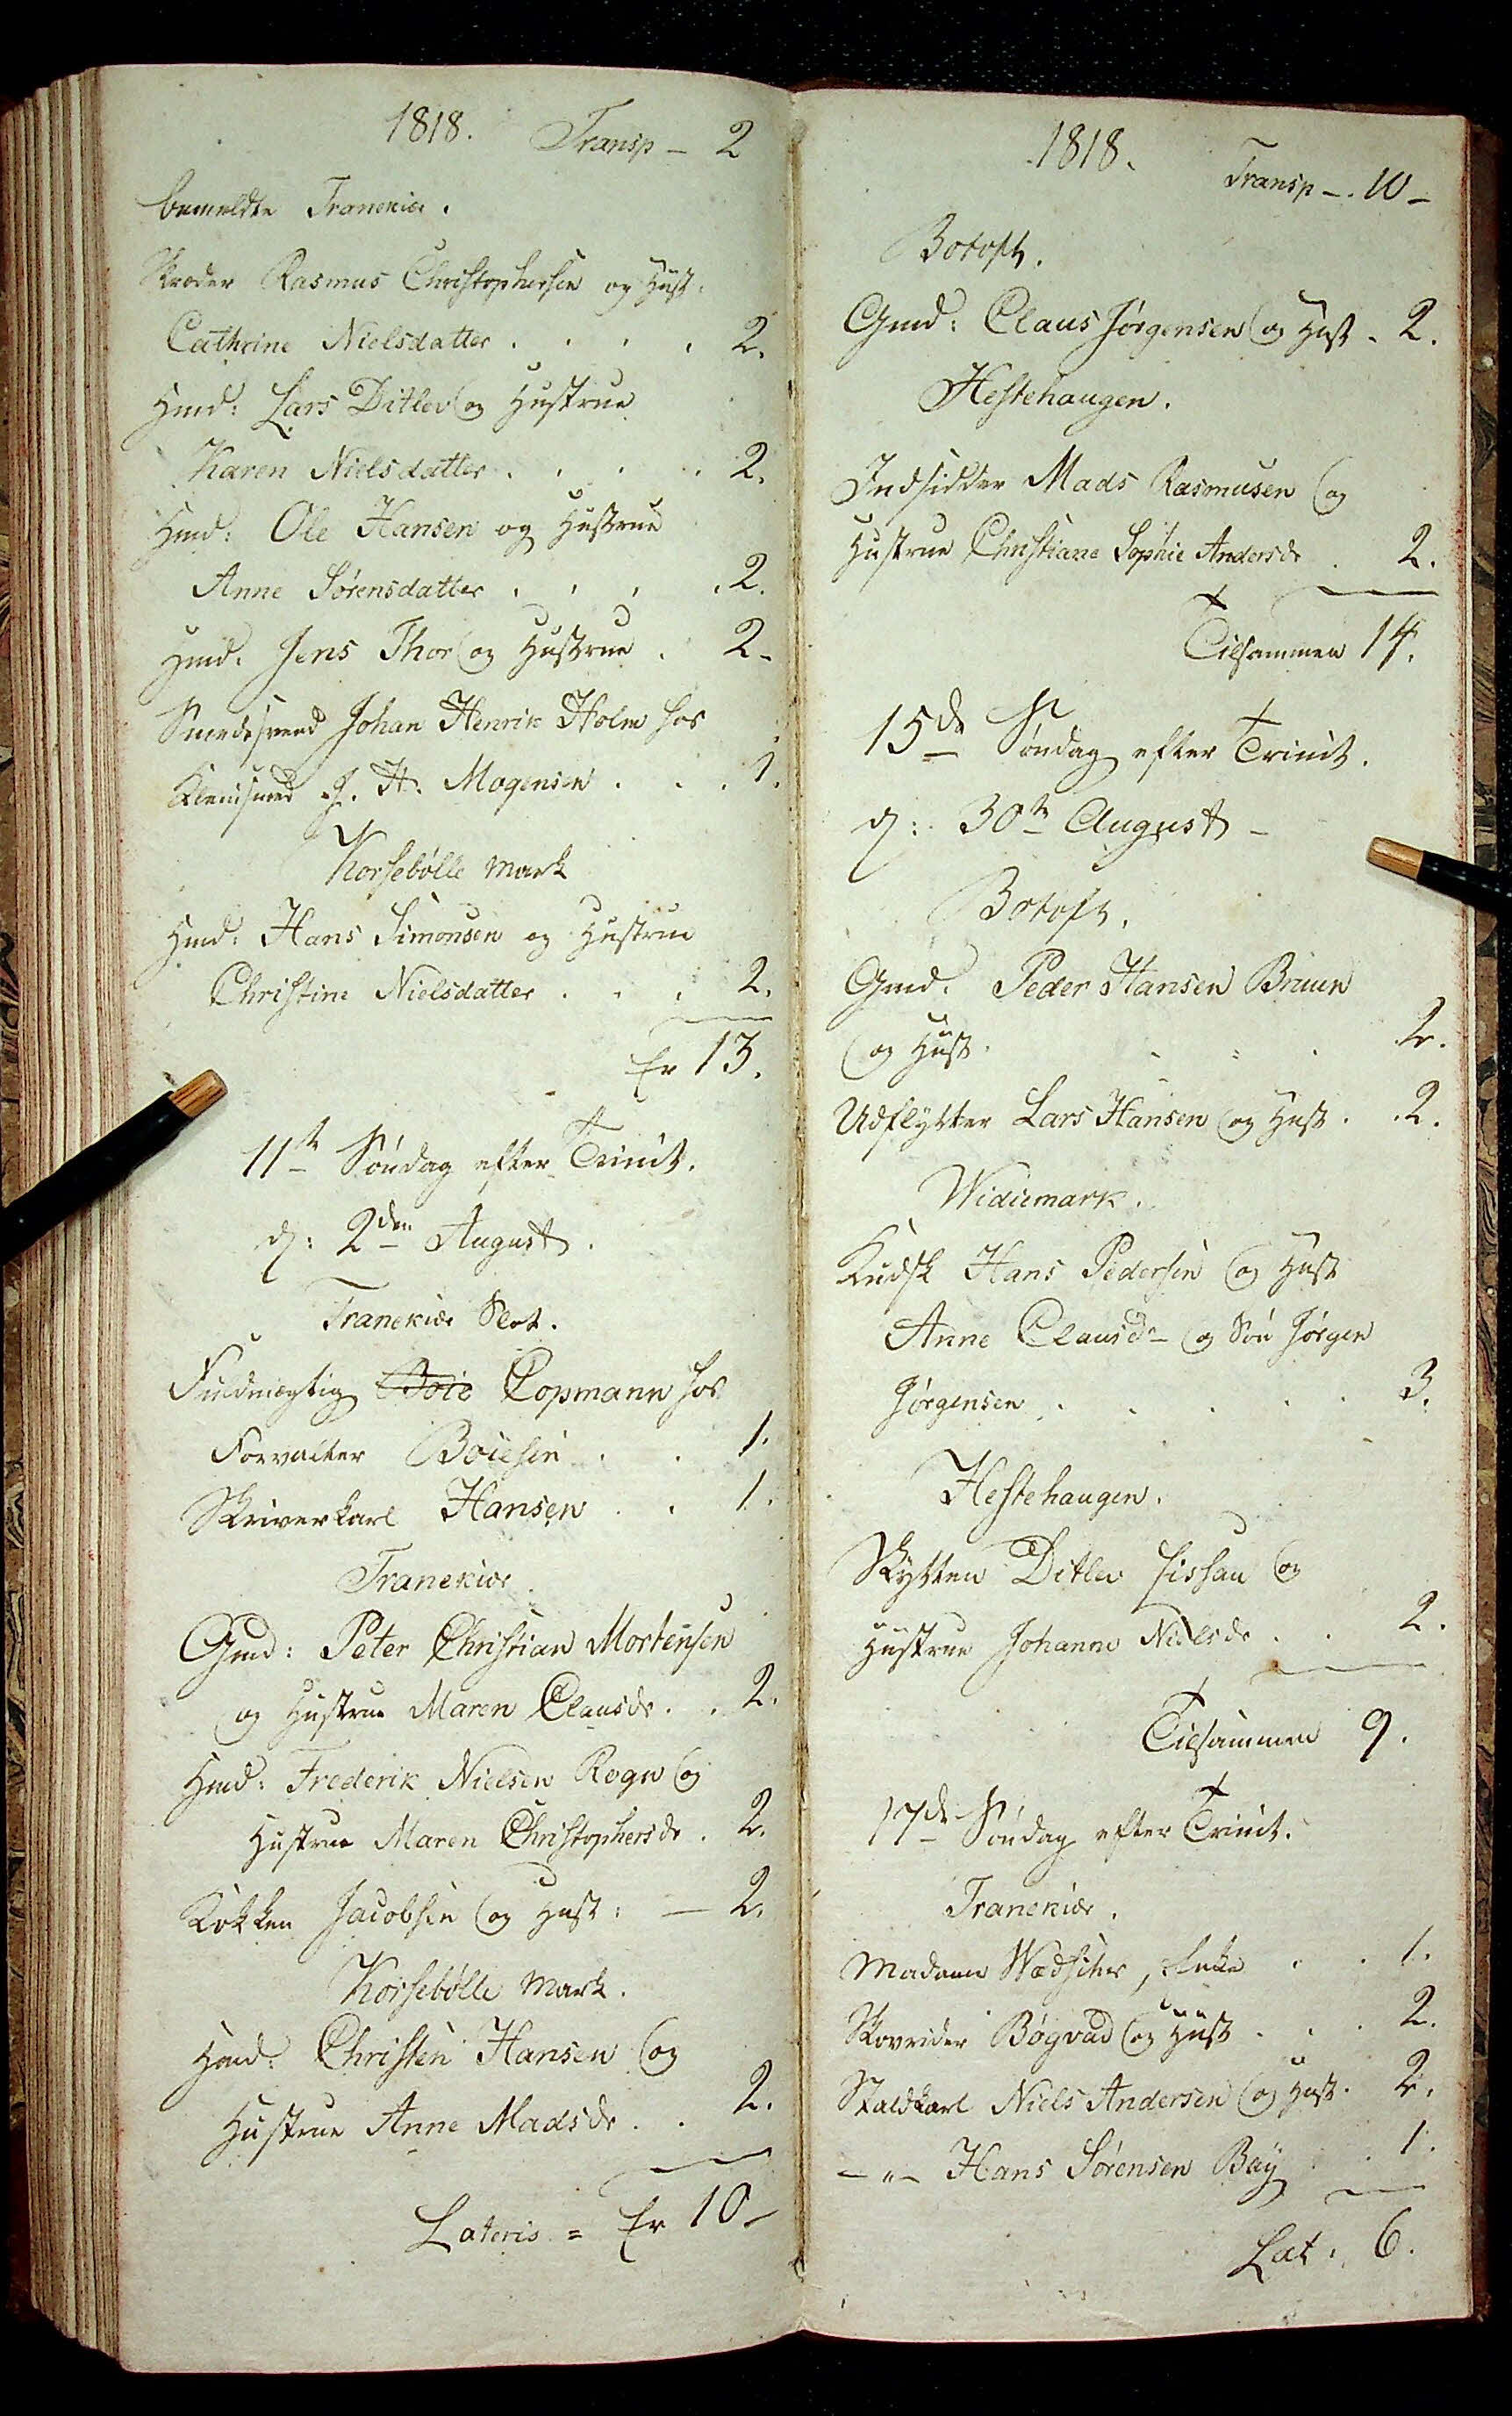1818.
Transp - 2
bemeldte Tranekiær.
Skræder Rasmus Christophersen og Hust:
Cathrine Nielsdatter . . . . 2.
Hmd: Lars Ditlev og Hustrue
Karen NielsDatter . . . . 2.
Hmd: Ole Hansen og Hustrue
Anne Sørensdatter . . . 2.
Hmd: Jens Thor og Hustrue . 2.
Smedsvend Johan Henrik Holm hos
Kleinsmed J. H. Mogensen . . .  1.
Korsebølle Mark
Hmd: Hans Simonsens og Hustrue
Christine Nielsdatter . . . 2.
Er 13.
11te Søndag efter Trinit.
d: 2den August
Tranekiær Slot.
Fuldmægtig Boie Copmann hos
Forvalter Boiesen . . 1.
Skriverkarl Hansen . . 1.
Tranekiær
Gmd: Peter Christian Mortensen
og Hustrue Maren Clausdr . . 2.
Hmd: Frederik Nielsen Rogn og
Hustrue Maren Christophersdr . 2.
Kokken Jacobsen og Hust: - 2.
Korsebølle Mark.
Hmd: Christen Hansen og
Hustrue Anne Madsdr . . 2.
Lateris = Er 10-
1818.
Transp - . 10-
Botoft..
Gmd: Claus Jørgensen og Hust . 2.
Hestehaugen.
Indsidder Mads Rasmusen og
Hustrue Christiane Sophie Andersdr . 2.
Tilsammen 14.
15de Søndag efter Trinit.
d: 30te August
Botoft.
Gmd. Peder Hansen Bruun
og Hust . . . . 2.
Udflytter Lars Hansen og Hust . 2.
Widiemark.
Kudsk Hans Pedersen og Hust
Anne Clausdr og Søn Jørgen
Jørgensen . . . . . 3.
Hestehaugen.
Skytten Ditlev Lissau og
Hustrue Johanne Nielsdr . .2.
Tilsammen 9.
17de Søndag efter Trinit.
Tranekiær.
Madame Wædscher, Enke . . 1.
Skovrider Bøgvad og Hust . . . 2.
Staldkarl Niels Andersen og Hust . 2.
-"- Hans Sørensen Bay . . 1.
Lat: 6.
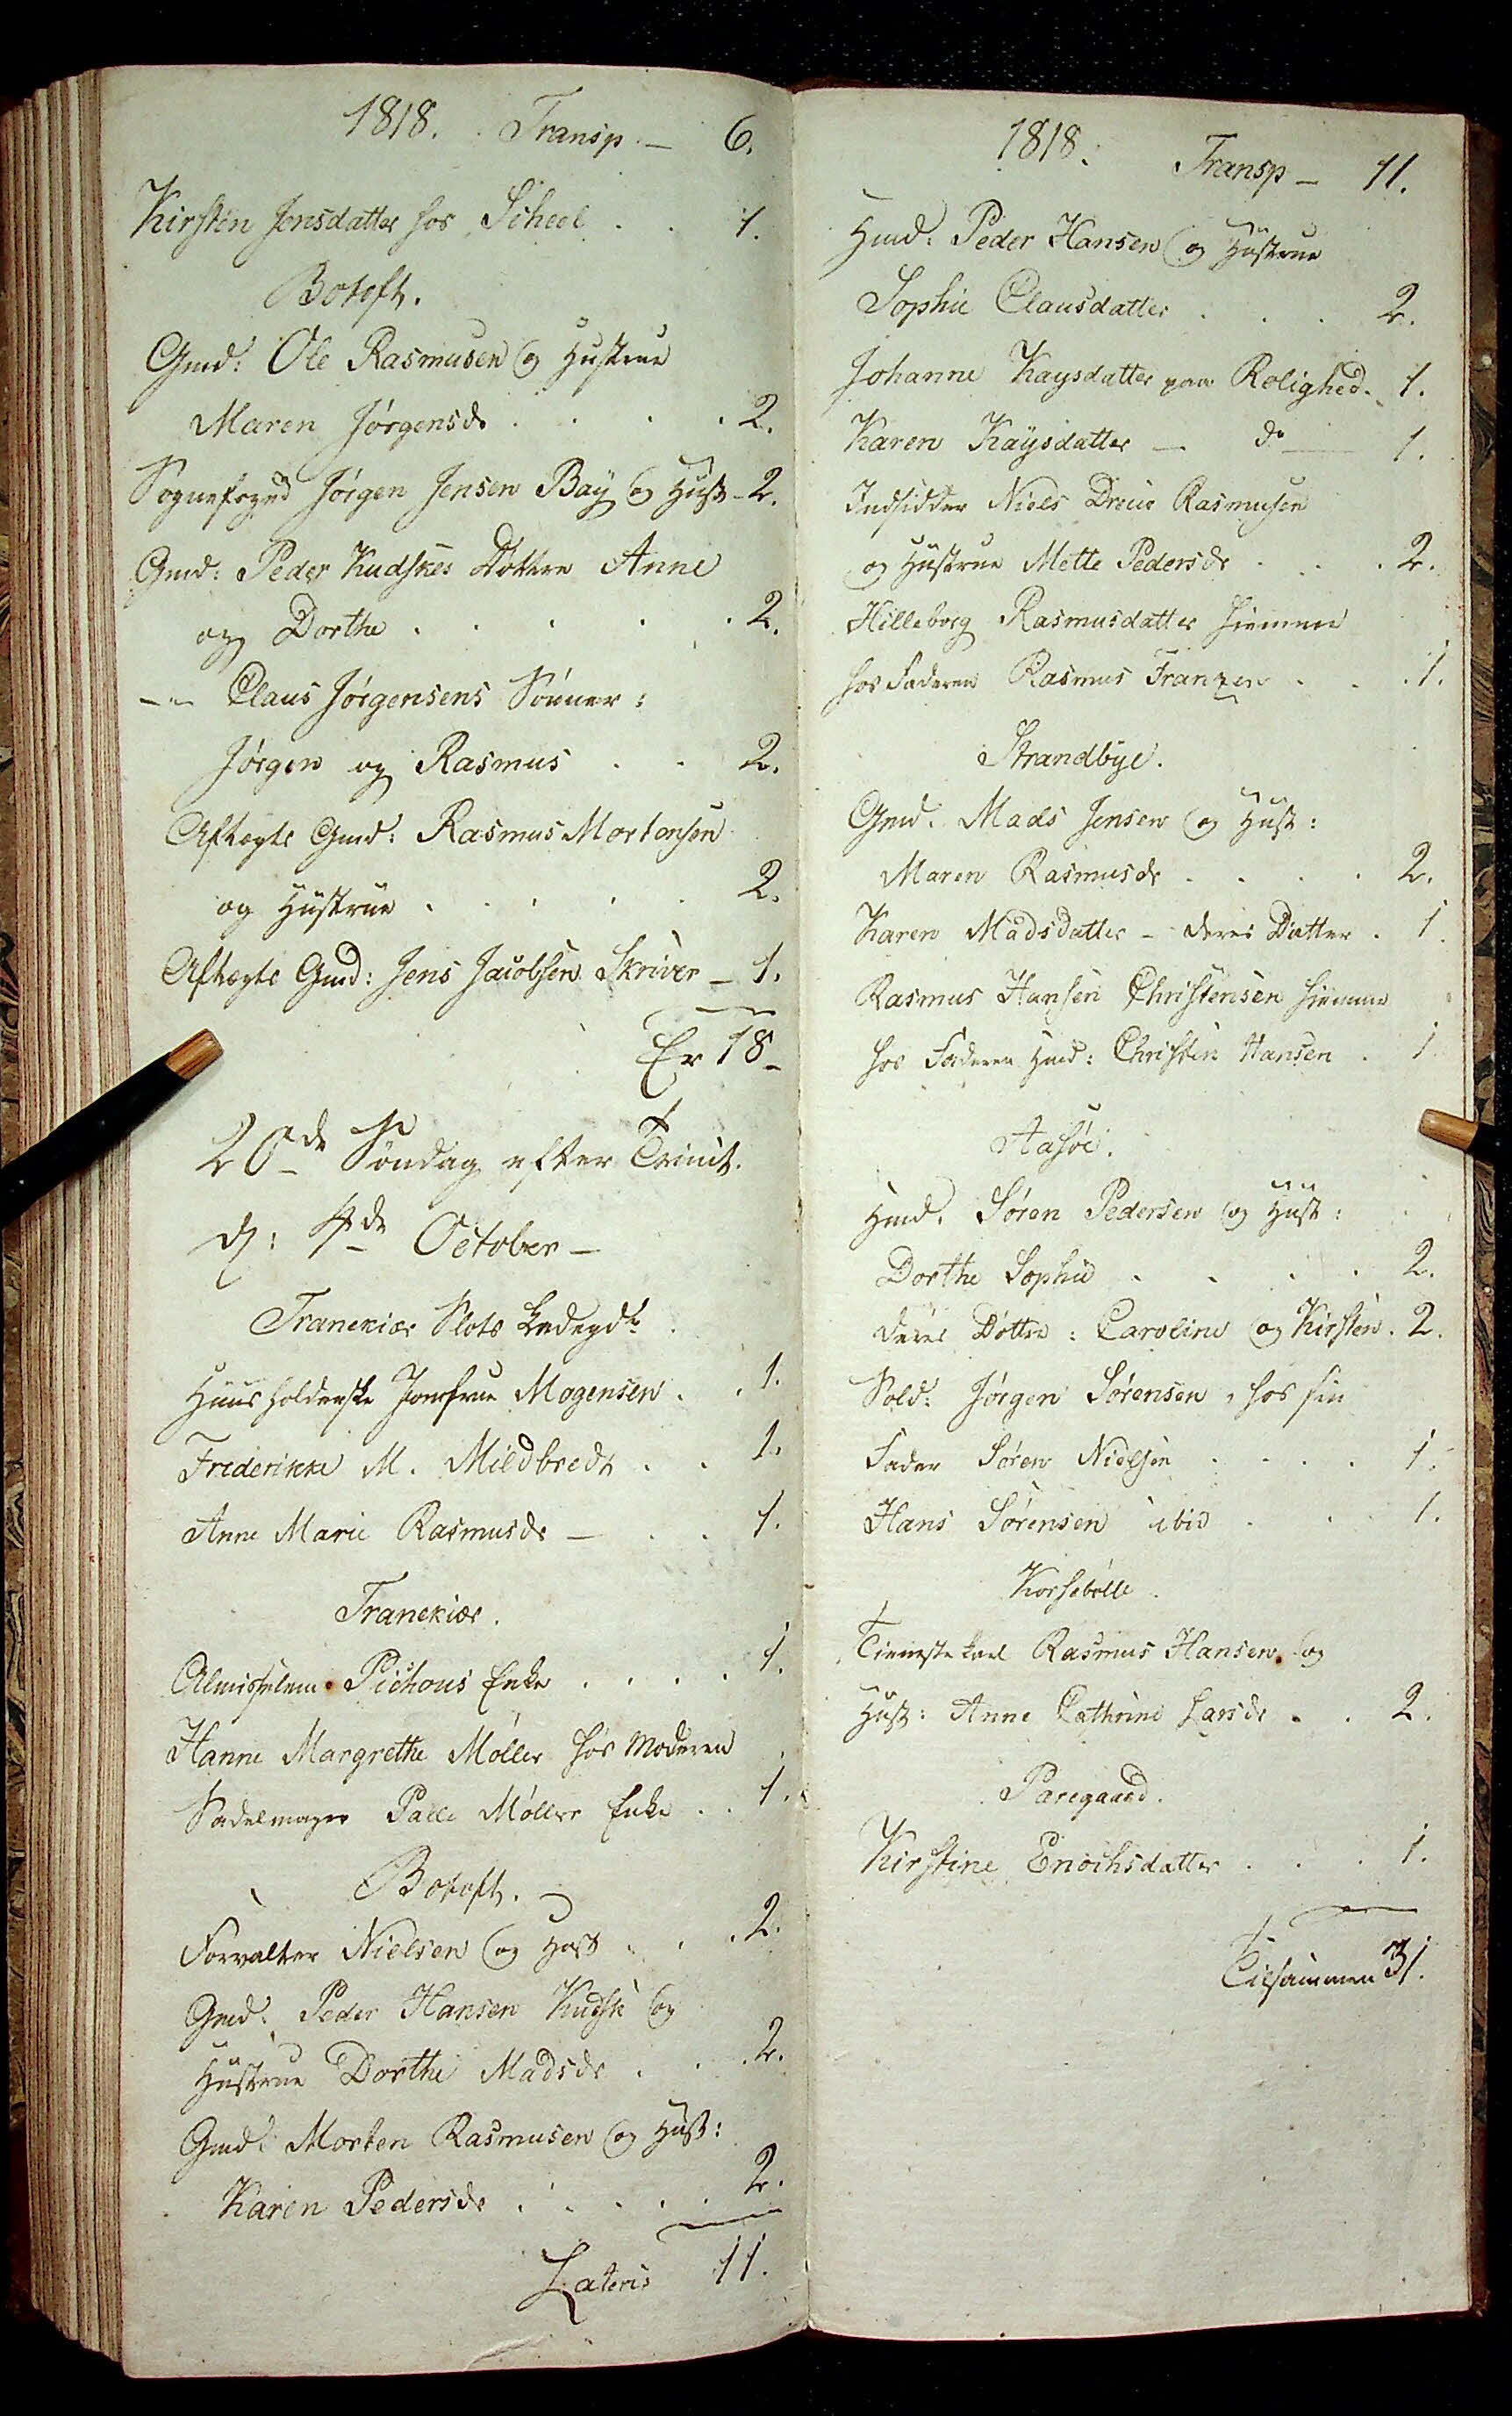1818.
Transp - 6.
Kirsten Jensdatter hos Scheel . . 1.
Botoft.
Gmd: Ole Rasmusen og Hustrue
Maren Jørgensdr . . . . 2.
Sognefoged Jørgen Jensen Bay og Hustrue - 2.
Gmd: Peder Kudskes Døttre Anne
og Dorthe . . . . . 2.
-"- Claus Jørgensens Sønner:
Jørgen og Rasmus . . 2.
Aftægts Gmd: Rasmus Mortensen
og Hustrue . . . . . 2.
Aftægts Gmd: Jens Jacobsens Skriver - 1.
Er 18.
20de Søndag efter Trinit.
d: 4de October-
Tranekiær Slots Ladegdr
Huusholderske Jomfrue Mogensen . . 1.
Friderikke M. Mildbredt . . 1.
Anne Marie Rasmusdr - . . 1.
Tranekiær.
Almisselem Pichous Enke . . . . 1.
Hanne Margrethe Møller hos Moderen
Sadelmager Palle Møllers Enke . .1.
Botoft.
Forvalter Nielsen og Hust . . . 2.
Gmd: Peder Hansen Kudsk og
Hustrue Dorthe Madsdr . . . 2.
Gmd: Morten Rasmusen og Hust:
Karen Pedersdr . . . . . 2.
Lateris 11.
1818.
Transp - 11.
Hmd: Peder Hansens og Hustrue
Sophie Clausdatter . . . 2.
Johanne Kaysdatter paa Rolighed . 1.
Karen Kaysdatter - do - 1.
Indsidder Niels Dreier Rasmusen
og Hustrue Mette Pedersdr . . . 2.
Hilleborg Rasmusdatter hiemme
hos Faderen Rasmus Franzen . . . 1.
Strandbye.
Gmd: Mads Jensen og Hust:
Maren Rasmusdr . . . . 2.
Karen Madsdatter - deres Datter . 1.
Rasmus Hansen Christensen hiemme
hos Faderen Hmd: Christen Hansen . 1.
Aasøe
Hmd. Søren Pedersen og Hust:
Dorthe Sophie . . . . 2.
deres Døttre: Caroline og Kirsten . 2.
Sold: Jørgen Sørensen, hos sin
Fader Jøren Nielsen . . . . 1.
Hans Sørensen ibid . . 1.
Korsebølle
Tienestekarl Rasmus Hansen og
Hust: Anne Cathrine Larsdr . . 2.
Paregaard.
Kirstine Enochsdatter . . . 1.
Tilsammen 31.